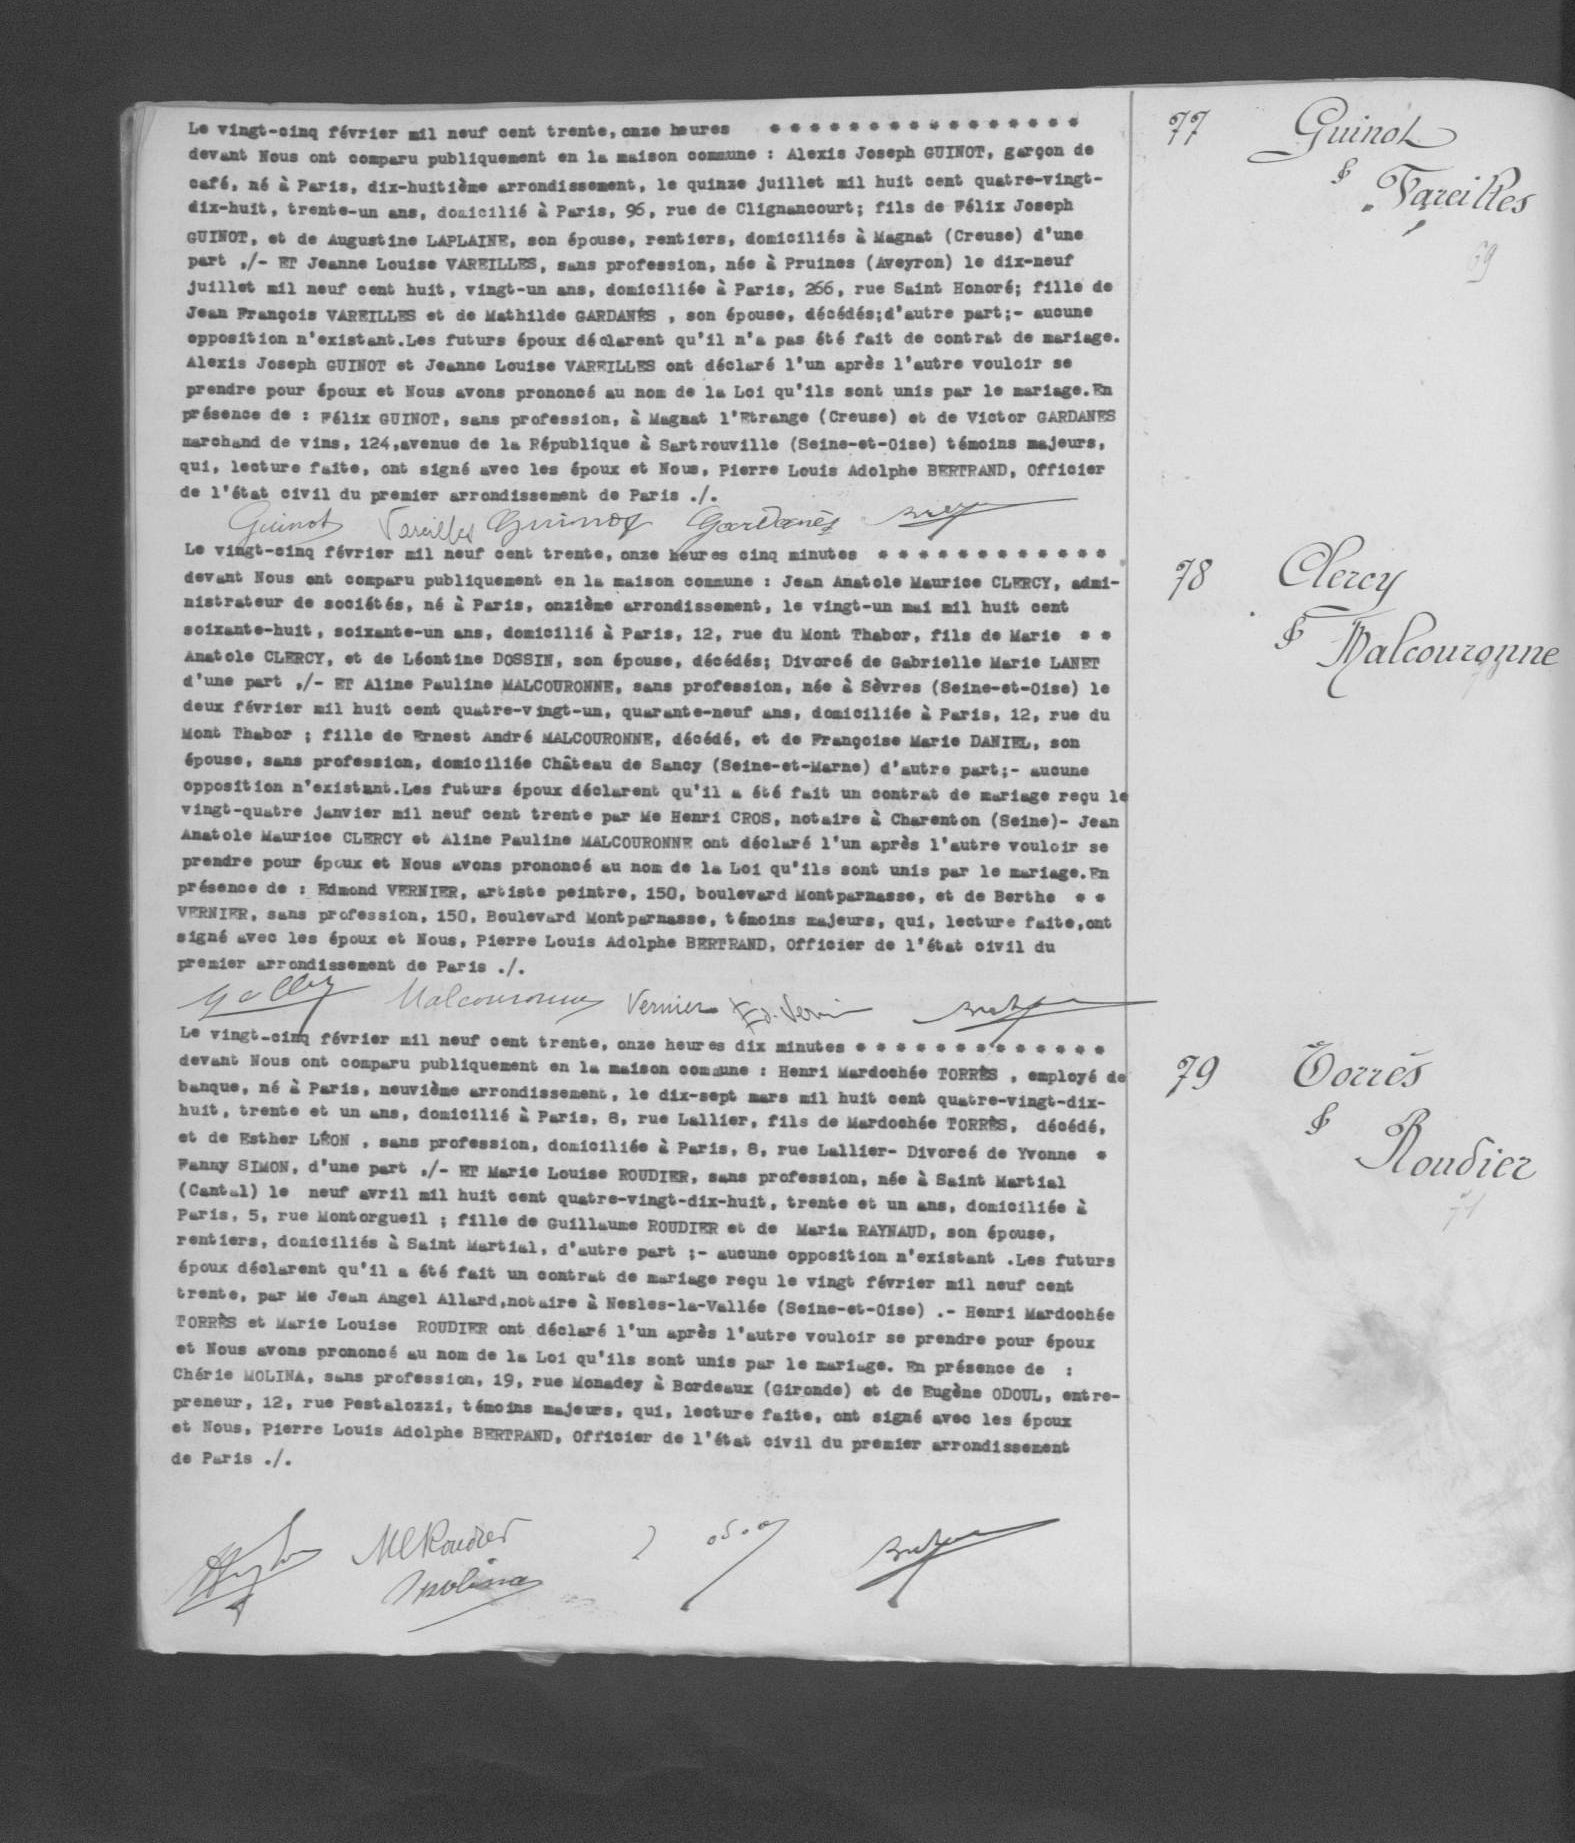Le vingt-cinq février mil neuf cent trente, onze heures ****
devant Nous ont comparu publiquement en la maison commune: Alexis Joseph GUINOT, garçon de
café, né à Paris, dix-huitième arrondissement, le quinze juillet mil huit cent quatre-vingt-
dix-huit, trente-un ans, domicilié à Paris, 96, rue de Clignancourt; fils de Félix Joseph
GUINOT, et de Augustine LAPLAINE, son épouse, rentiers, domiciliés à Magnat (Creuse) d'une
part ,/-ET Jeanne Louise VAREILLES, sans profession, née à Pruines (Aveyron) le dix-neuf
juillet mil neuf cent huit, vingt-un ans, domiciliée à Paris, 266, rue Saint Honoré; fille de
Jean François VAREILLES et de Mathilde GARDANES, son épouse, décédés; d'autre part ;-aucune
opposition n'existant. Les futurs époux déclarent qu'il n'a pas été fait de contrat de mariage.
Alexis Joseph GUINOT et Jeanne Louise VAREILLES ont déclaré l'un après l'autre vouloir se
prendre pour époux et Nous avons prononcé au nom de la Loi qu'ils sont unis par le mariage. En
présence de: Félix GUINOT, sans profession, à MAgnat l'Etrange (Creuse) et de Victor GARDANES
marchand de vins, 124, avenue de la République à Sartrouville (Seine-et-Oise) témoins majeurs,
qui, lecture faite, ont signé avec les époux et Nous, Pierre Louis Adolphe BERTRAND, Officier
de l'état civil du premier arrondissement de Paris ./.
Le vingt-cinq février mil neuf cent trente, onze heures cinq minutes ****
devant Nous ont comparu publiquement en la maison commune: Jean Anatole Maurice CLERCY ,, admi-
nistrateur de sociétés, né à Paris, onzième arrondissement, le vingt-un mai mil huit cent
soixante-huit, soixante-un ans, domicilié à Paris, 12, rue du Mont Thabor, fils de Marie * *
Anatole CLERCY, et de Léontine DOSSIN, son épouse, décédés; Divorcé de Gabrielle Marie LANET
d'une part ,-/ ET Aline Pauline MALCOURONNE, sans profession, née à Sèvres (Seine-et-Oise) le
deux février mil huit cent quatre-vingt-un, quarante-neuf ans, domiciliée à Paris, 12, rue du
Mont Thabor; fille de Ernest André MALCOURONNE, décédé, et de Françoise Marie DANIEL son
épouse, sans profession, domiciliée Château de Sancy (Seine-et-Marne) d'autre part ;-aucune
opposition n'existant. Les futurs époux déclarent qu'il a été fait un contrat de mariage reçu le
vingt-quatre janvier mil neuf cent trente par Me Henri CROS, notaire à Charenton (Seine)-Jean
Anatole Maurice CLERCY et Aline Pauline MALCOURONNE ont déclaré l'un après l'autre vouloir se
prendre pour époux et Nous avons prononcé au nom de la Loi qu'ils sont unis par le mariage. En
présence de: Edmond VERNIER, artiste peintre, 150, boulevard Montparnasse, et de Berthe * *
VERNIER, sans profession, 150, Boulevard Montparnasse, témoins majeurs, qui, lecture faite, ont
signé avec les époux et Nous, Pierre Louis Adolphe BERTRAND, Officier de l'état civil du
premier arrondissement de Paris ./.
Le vingt-cinq février mil neuf cent trente, onze heures dix minutes ****
devant Nous ont comparu publiquement en la maison commune: Henri Mardochée TORRES, employé de
banque, né à Paris, neuvième arrondissement, le dix-sept mars mil huit cent quatre-vingt-dix-
huit, trente et un ans, domicilié à Paris, 8, rue Lallier, fils de Mardochée TORRES, décédé,
et de Esther LEON, sans profession, domiciliée à Paris, 8, rue Lallier-Divorcé de Yvonne *
Fanny SIMON, d'une part ,/-ET Marie Louise ROUDIER, sans profession, née à Saint Martial
(Cantal) le neuf avril mil huit cent quatre-vingt-dix-huit, trente et un ans, domiciliée à
Paris, 5, rue Montorgueil; fille de Guillaume ROUDIER et de Marie RAYNAUD, son épouse,
rentiers, domiciliés à Saint Martial, d'autre part ;-aucune opposition n'existant. Les futurs
époux déclarent qu'il a été fait un contrat de mariage reçu le vingt février mil neuf cent
trente, par Me Jean Angel Allard, notaire à Nesles-la-Vallée (Seine-et-Oise) .-Henri Mardochée
TORRES et Marie Louise ROUDIER ont déclaré l'un après l'autre vouloir se prendre pour époux
et Nous avons prononcé au nom de la Loi qu'ils sont unis par le mariage. En présence de:
Chérie MOLINA, sans profession, 19, rue Monadey à Bordeaux (Gironde) et de Eugène ODOUL, entre-
preneur, 12, rue Pestalozzi, témoins majeurs, qui, lecture faite, ont signé avec les époux
et Nous, Pierre Louis Adolphe BERTRAND, Officier de l'état civil du premier arrondissement
de Paris ./.
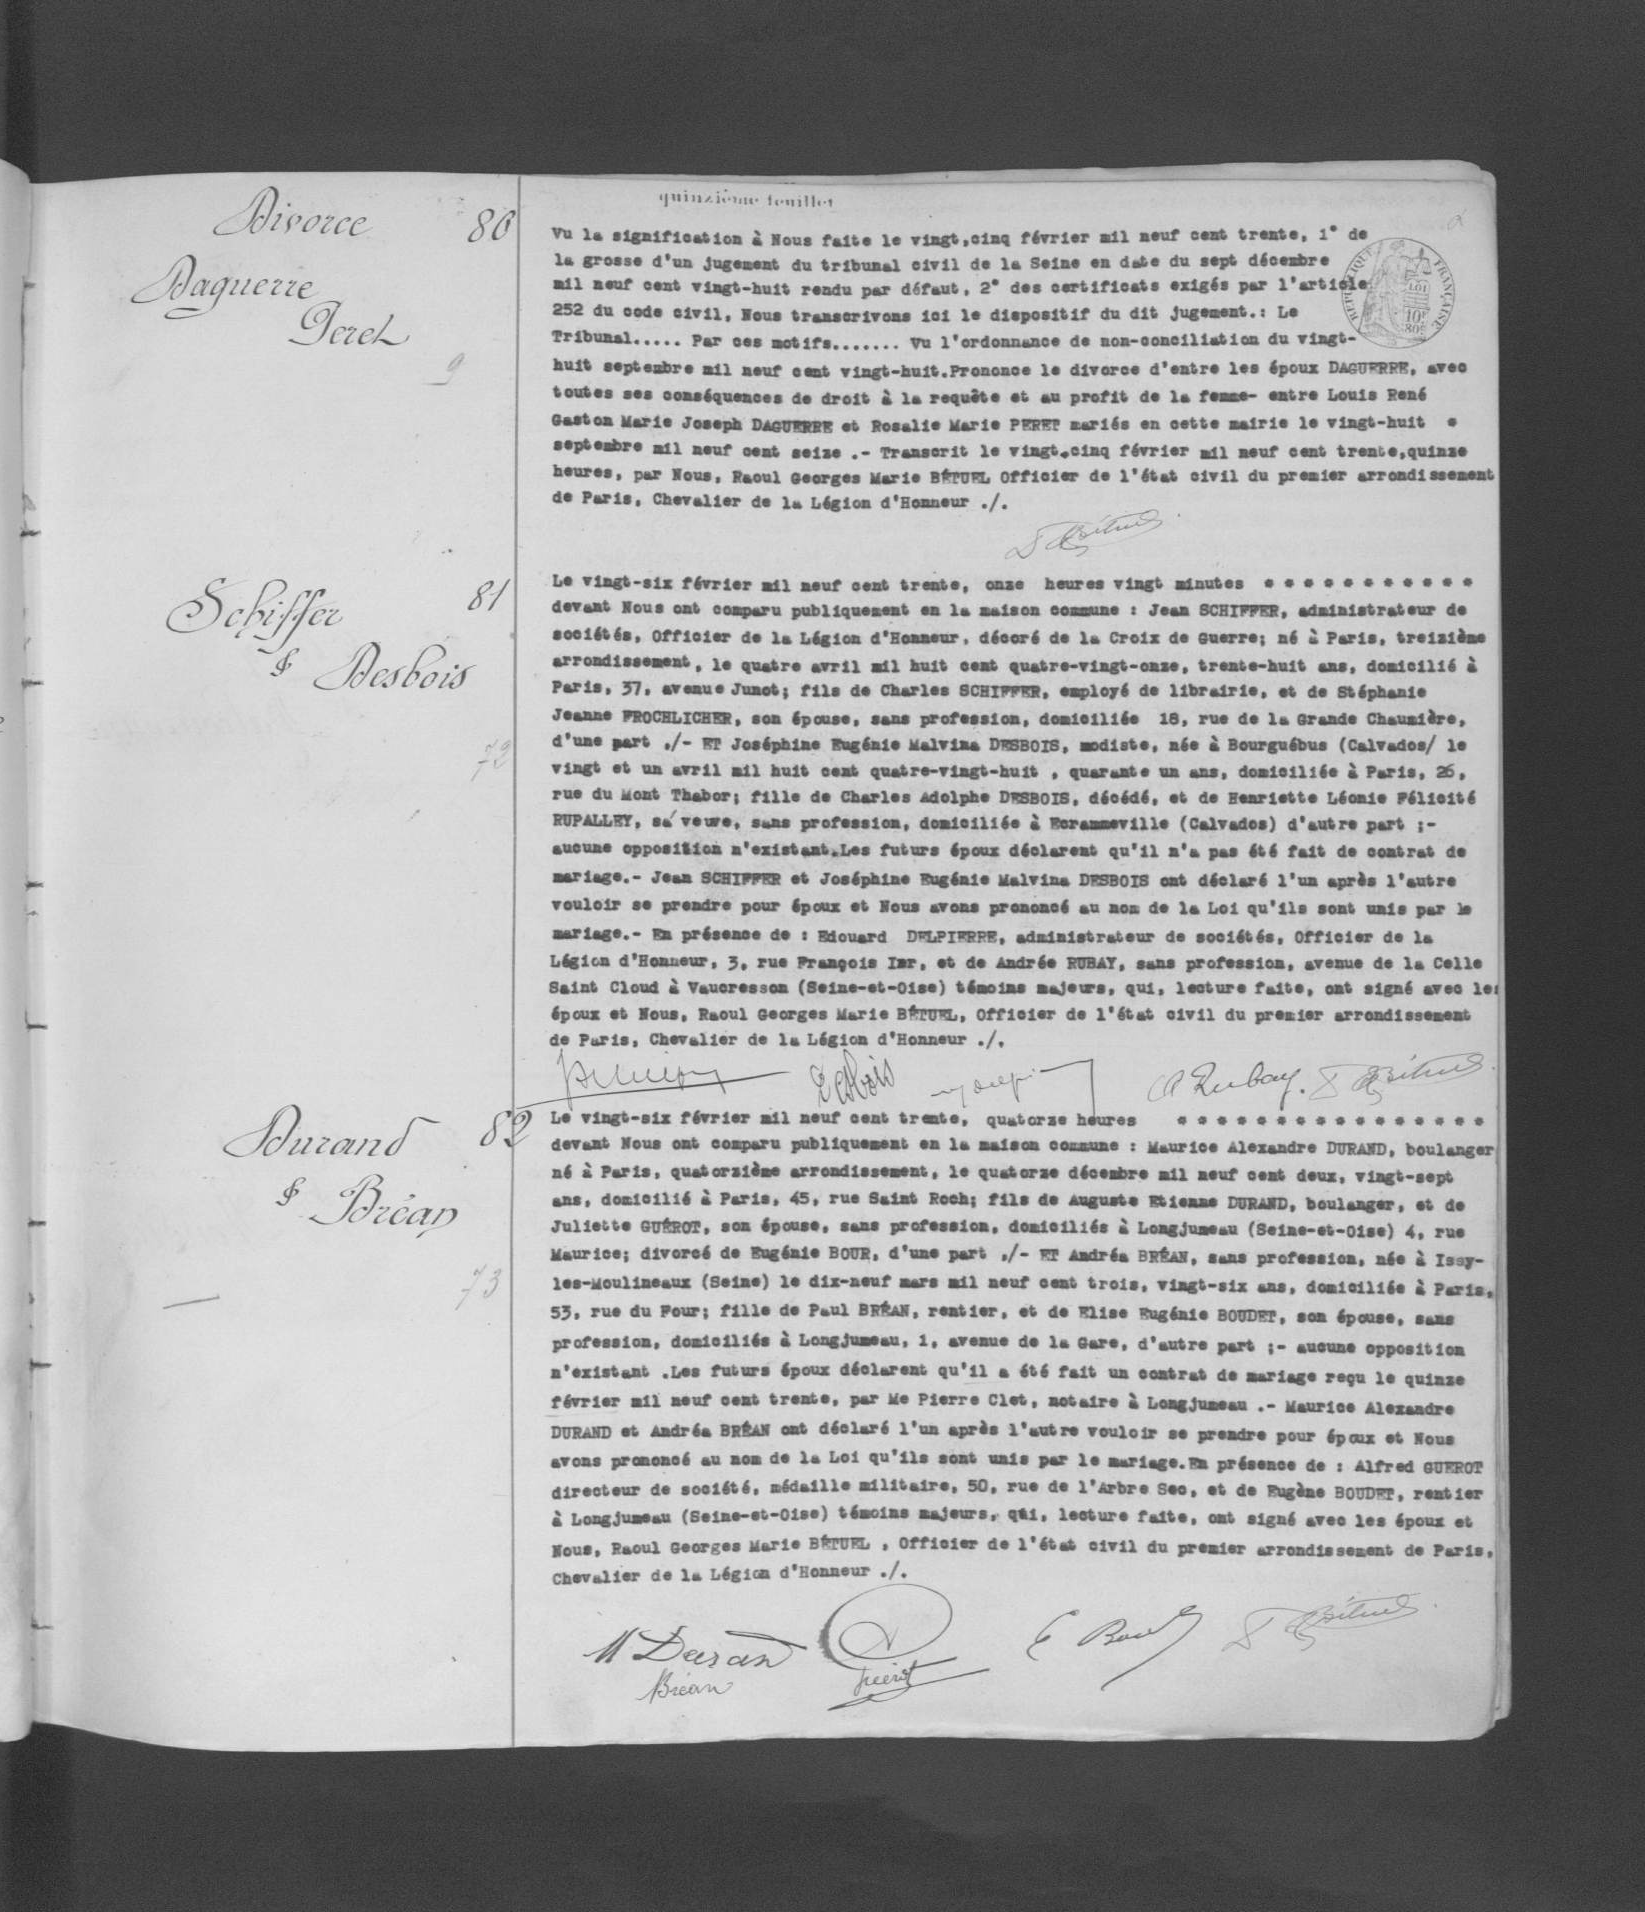Vu la signification à Nous faite le vingt, cinq février mil neuf cent trente, 1° de
la grosse d'un jugement du tribunal civil de la Seine en date du sept décembre
mil neuf cent vingt-huit rendu par défaut, 2° des certificat exigés par l'article
252 du code civil, Nous transcrivons ici le dispositif du dit jugement.: Le
Tribunal ..... Par ces motifs ..... Vu l'ordonnanc de non-conciliation du vingt-
huit septembre mil neuf cent vingt-huit. Prononce le divorce d'entre les époux DAGUERRE, avec
toutes ses conséquences de droit à la requête et au profit de la femme-entre Louis René
Gaston Marie Joseph DAGUERRE et Rosalie Marie PERET mariés en cette mairie le vingt-huit *
septembre mil neuf cent seize .-Transcrit le vingt cinq février mil neuf cent trente, quinze
heures, par Nous, Raoul Georges Marie BETUEL Officier de l'état civil du premier arrondissement
de Paris, Chevalier de la Légion d'Honneur ./.
Le vingt-six février mil neuf cent trente, onze heures vingt minutes ****
devant Nous ont comparu publiquement en la Maison commune: Jean SCHIFFER, administrateur de
sociétés, Officier de la Légion d'Honneur, décoré de la croix de Guerre; né à Paris, treizième
arrondissement, le quatre avril mil huit cent quatre-vingt-onze, trente-huit ans, domicilié à
Paris, 37, avenue Junoy; fils de Charles SCHIFFER, employé de librairire, et de Stéphanie
Jeanne FROCHLICHER, son épouse, sans profession, domiciliée 18, rue de la Grande Chaumière,
d'une part ,/-ET Joséphine Eugénie Malvina DESBOIS, modiste, née à Bourguébus (Calvados/ le
vingt et un avril mil huit cent quatre-vingt-huit, quarante un ans, domiciliée à PAris, 26,
rue du Mont Thabor; fille de Charles Adolphe DESBOIS, décédé, et de Henriette Léonie Félicité
RUPALLEY, sa veuve, sans profession, domiciliée à Ecracrammeville (Calvados) d'autre part ;-
aucune opposition n'existant. Les futurs époux déclarent qu'il n'a pas été fait de contrat de
mariage .-Jean SCHIFFER et Joséphine Eugénie Malvina DESBOIS ont déclaré l'un après l'autre
vouloir se prendre pour époux et Nous avons prononcé au nom de la Loi qu'ils sont unis par le
mariage .-En présence de: Edouard DELPIERRE, administrateur de sociétés, Officier de la
Légion d'Honneur, 3, rue François Ier, et de André RUBAY, sans profession, avenue de la Celle
Saint Clourd à Vaucresson (Seine-et-Oise) témoins majeurs, qui, lecture faite, ont signé avec les
époux et Nous, Raoul Georges Marie BETUEL, Officier de l'état civil du premier arrondissement
de Paris, Chevalier de la Légion d'Honneur ./.
Le vingt-six février mil neuf cent trente, quatorze heures ****
devant Nous ont comparu publiquement en la maison commune: Maurice Alexandre DURAND, boulanger
né à Paris, quatorzième arrondissement, le quatorze décembre mil neuf cent deux, vingt-sept
ans, domicilié à Paris, 45, rue Saint Roch; fils de Auguste Etienne DURAND, boulanger, et de
Juliette GUEROT, son épouse, sans profession, domiciliés à Longjumeau (Seine-et-Oise) 4, rue
Maurice; divorcé de Eugénie BOUR, d'une part ,/-ET Andréa BREAN, sans profession, née à Issy-
les-Moulineaux (Seine) le dix-neuf mars mil neuf cent trois, vingt-six ans, domiciliée à Paris,
53, rue du Four; fille de Paul BREAN, rentier, et de Elise Eugénie BOUDET, son épouse, sans
profession, domiciliés à Longjumeau, 1, avenue de la Gare, d'autre part ;-aucune opposition
n'existant. Les futurs époux déclarent qu'il a été fait un contrat de mariage reçu le quinze
février mil neuf cent trente, par Me Pierre Clet, notaire à Longjumeau .-Maurice Alexandre
DURAND et Andréa BREAN ont déclaré l'un après l'autre vouloir se prendre pour époux et Nous
avons prononcé au nom de la Loi qu'ils sont unis par le mariage. En présence de: Alfred GUEROT
directeur de société, médaille militaire, 50, rue de l'Arbre Sec, et de Eugène BOUDET, rentier
à Longjumeau (Seine-et-Oise) témoins majeurs, qui, lecture faite, ont signé avec les époux et
Nous, Raoul Georges Marie BETUEL, Officier de l'état civil du premier arrondissement de Paris,
Chevalier de la Légion d'Honneur ./.